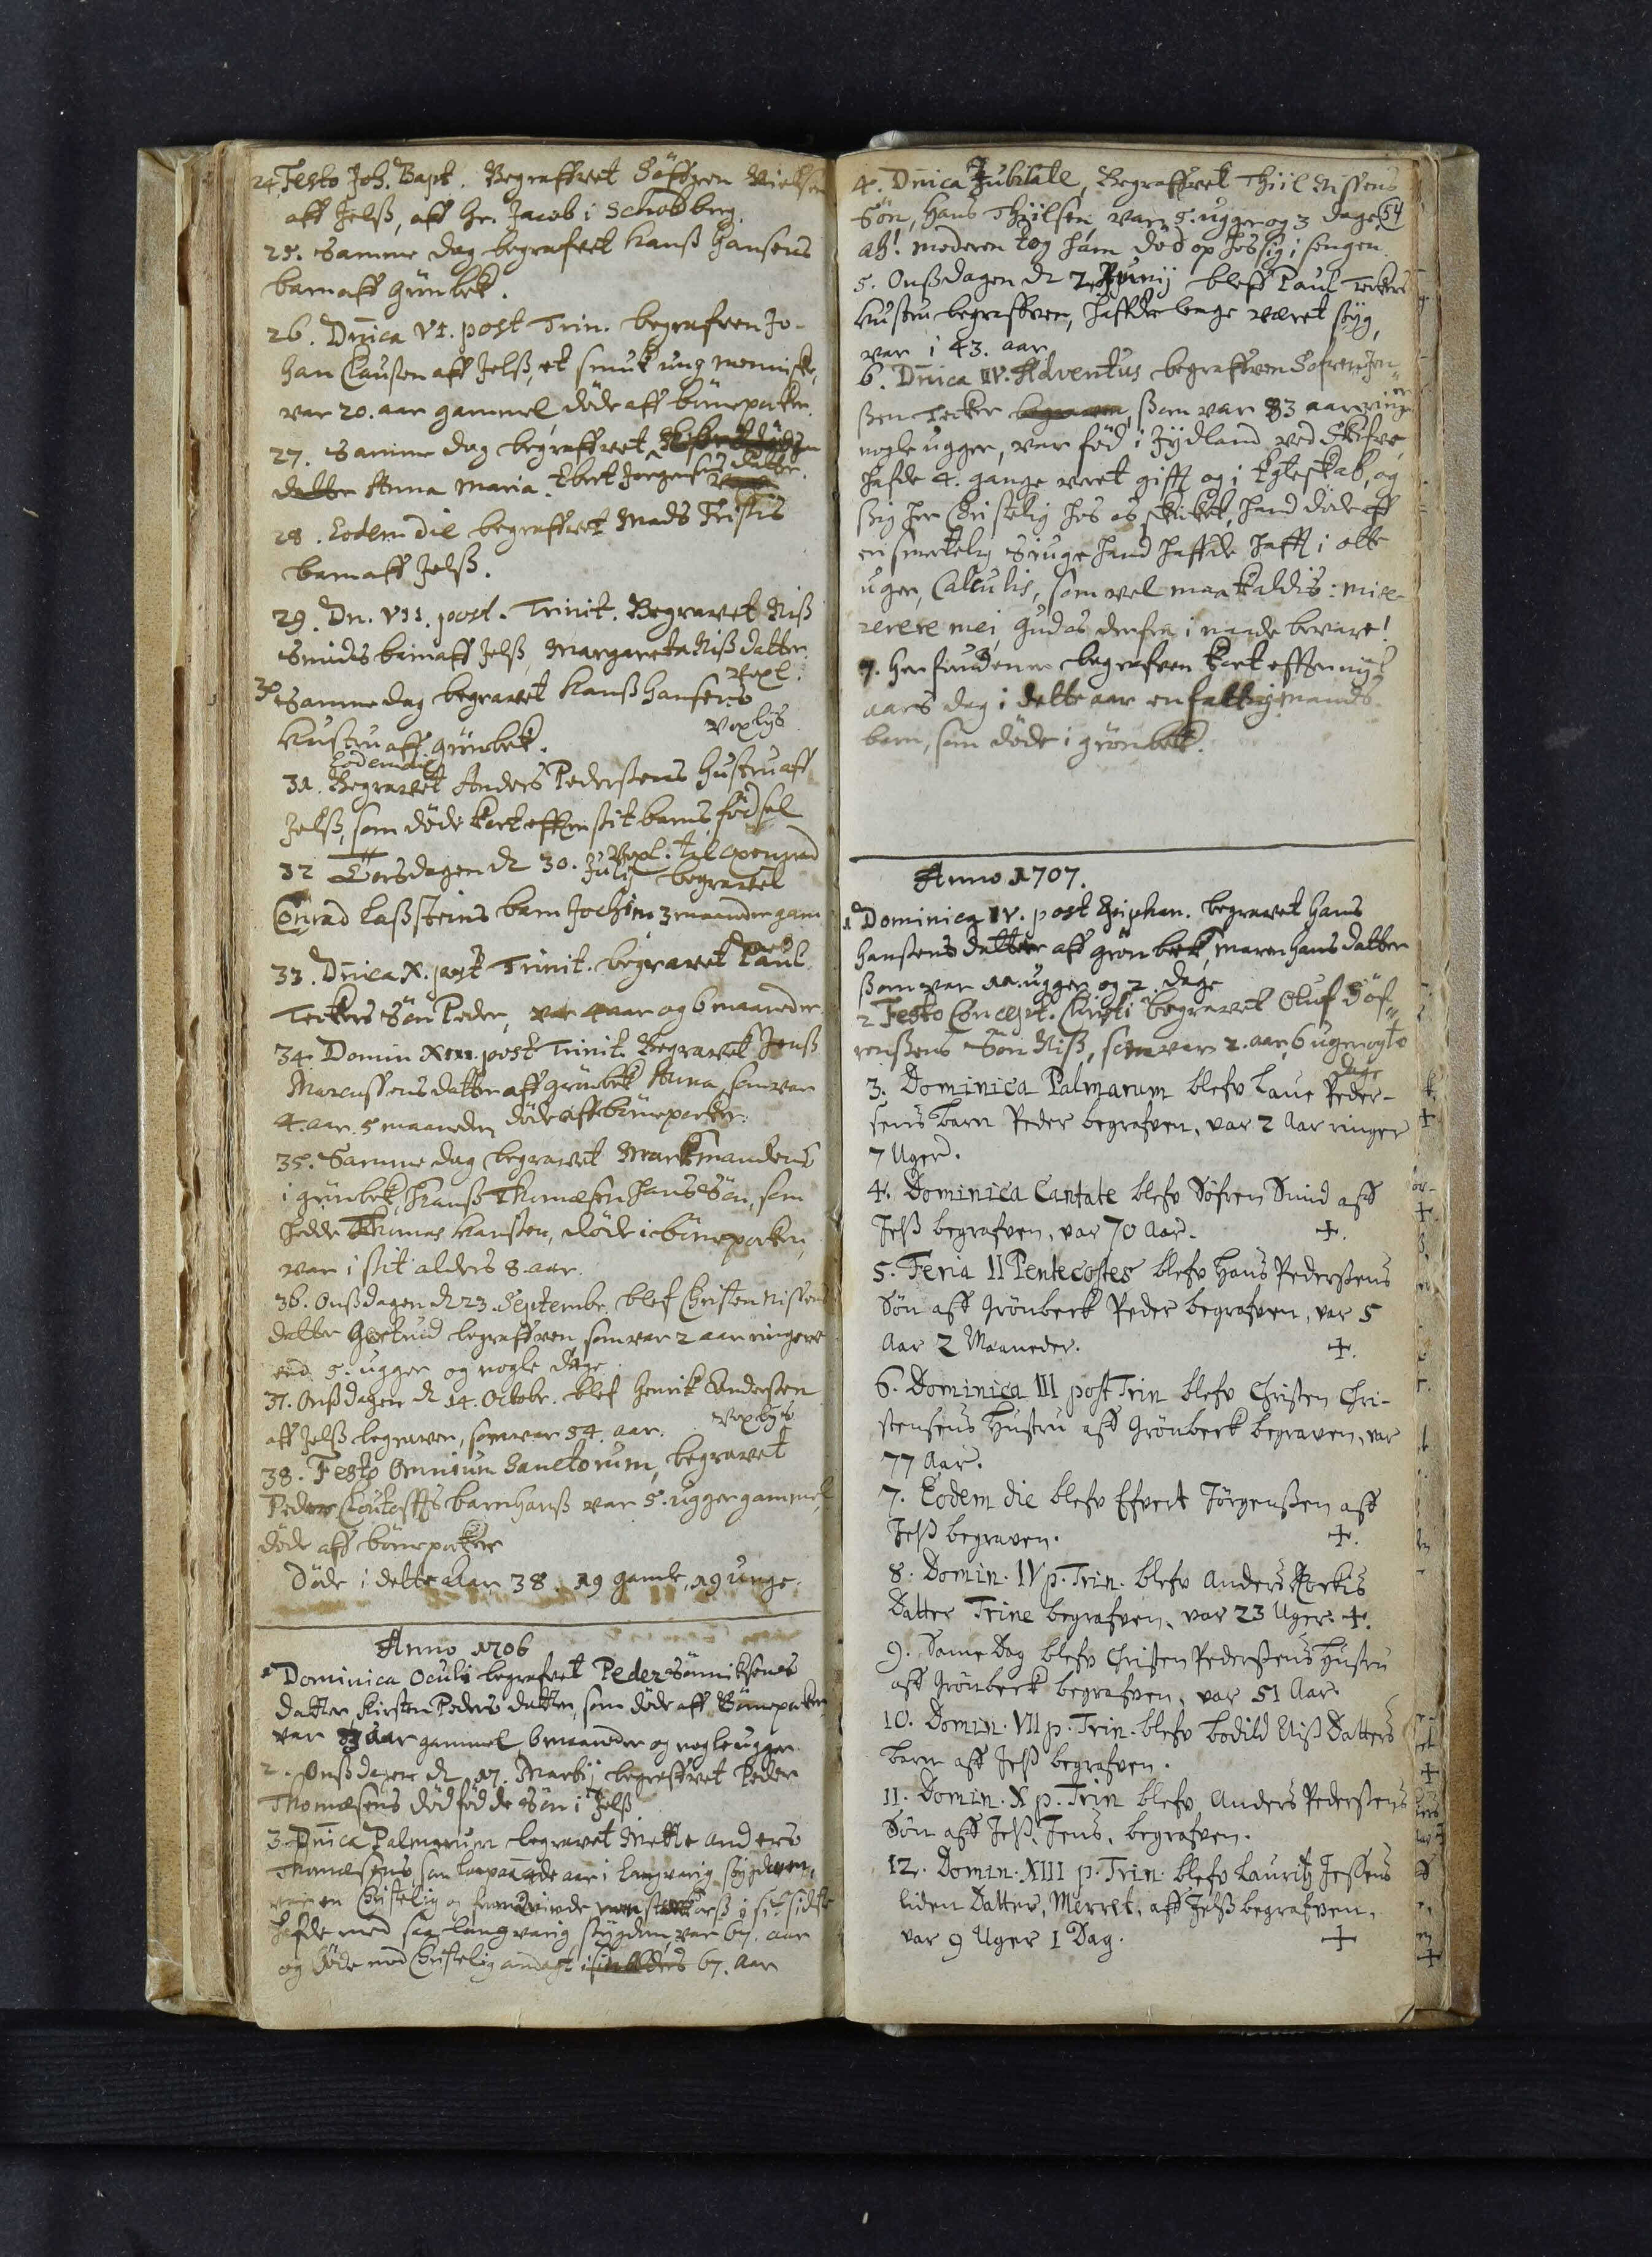24. Festo Joh. Bapt. Begraffvet Søffren Nielsen
aff Jels, aff Hr. Jacob i Schodborg
25. Samme Dag begrafvet Hanss Hansens
barn aff Grønbek.
26. Dnica VI. post Trin. begrafven Jo¬
han Claussen aff Jelss, et smuk ung menniske,
var 20. aar gammel, døde aff børnepocker.
27. Samme dag begraffvet Ebert Jørgensens
datter Anna Maria. 
Ebert Jørgensens datter
28. Eodem die begraffvet Mads Frisis
barn aff Jelss.
29. Dn. VII. post Trinit. Begravet Niss
Smids barn aff Jelss, Margaretha Niss Datter.
Woxl.
30. Samme dag begravet Hanss Hansens
Hustru aff Grønbek.
Woxlys
Eodem die
31. begravet Anders Pederssens Hustru aff
Jelss, som døde kort effter sit barns fødsel
Woxl. Til Oxenvad
32. Torsdagen d 30. Julij begravet
Conrad Lassteins barn Jochim 3 maaneder gam
33. Dnica X. post Trinit. begravet Paul
Teckers søn Peder, var 4 aar og 6 maaneder
34. Domin XIII. post Trinit. begravet Jenss
Marcussens datter aff Grønbek Anna, som var
4. aar. 5 maaneder døde aff børnepocker
35. Samme dag begravet Markmandens 
i Grønbek, Hans Thomæsen hans Søn, som 
hedde Thomas Hansen, døde aff børnepocker,
var i ssit alders 8. aar
36. Onssdagen d 23. Septembr. blef Christen Nissens
datter Gertrud begraffven som var 2 aar ringere
end 5. ugger og nogle dage
37. Onssdagen d 14. Octobr. blef Henrik Anderssen
aff Jelss Begraven, som var 34. aar. Woxlys
38. Festo Omnium Sanctorum , begravet
Peder Cloutoffts barn Hanss var 5. ugger gammel,
døde aff børnepocker
Døde i dette Aar 38. 19 gamle, 19 unge
Anno 1706
1. Dominica Oculi begrafvet Peder Sønnicksens
datter, Kirsten Peders Datter, som døde aff Børnepocker,
var 3 Aar gammel 6 maaneder og nogle ugger
2. Onssdagen d 17. Martij begraffvet Peder
Thomæsens dødfødde søn i Jelss
3. Dnica Palmarum begravet Mette Anders
Thomæsens, som laa paa 4de aar i langvarig sygdom,
var en Christelig og from Qvinde men st##ess i sit sidste
hafde med saa langvarig sygdom, var 67. aar
og døde med Christelig andakt i sin alders 67. Aar.
54
4. Dnica Jubilate , Begraffvet Thiil Nissens
søn, Hans Thiilsen, var 5. ugger og 3 dage,
ah! Moderen tog ham død op hos sig i sengen.
5. Onssdagen d 2. Junij bleff Paul Teckers
Hustru begraffven, hafde lenge været syg,
var i 43. aar
6. Dnica IV. Adventus begraffven Søfren Jen¬
ssen Tecker ##, som var 83 aar ringer
nogle ugger, var fød i Jydland ved Skifve,
hafde 4. gange veret gifft og i Egteskab, og
ssig her Christelig hos os skicket, hand døde aff
en smertelig Siuge hand haffde haft i otte
uger, Caleulis, som vel maa kaldis: mise
rerere mei, Gud os derfra i naade bevare
7. Her funden## begrafven kort effter nyt
aars dag i dette aar en fattig mands
barn, som døde i Grønbek.
Anno 1707.
1. Dominica IV. post Epiphan. begravet Hans
Hanssens datter aff Grønbeck, Maren Hans datter
ssom var 11. ugger og 2. dage
2. Festo Concept. Christi begravet Oluf Søf¬
renssens Søn Niss, som var 2. aar, 6 uger og to
Dage
3. Dominica Palmarum blefv Laue Peder¬
sens barn Peder begrafven, var 2 Aar ringer
7 Uger.
4. Dominica Cantate blefv Søfren Smid aff
Jelss begrafven, var 70 Aar.
5. Feria II Pentecostes blefv Hans Pederssens
Søn aff Grønbeck Peder begrafven, var 5
Aar 2 Maaneder.
6. Dominica III post Trin blefv Christen Chri¬
stensens Hustru aff Grønbeck begraven, var
77 Aar.
7. Eodem die blefv Efvert Jørgenssen aff
Jels begraven.
8. Domin. IV p. Trin. blefv Anders Kockis
Datter Trine begrafven, var 23 Uger.
9. Same Dag blefv Christen Pederssens Hustru
aff Grønbeck begrafven, var 51 Aar.
10. Domin. VII p. Trin. blefv Bodild Niss Datters
barn aff Jels begrafven
11. Domin. X p. Trin. blefv Anders Pedersens
Søn aff Jels, Jens, begrafen.
12. Domin. XIII p. Trin. blefv Lauritz Jessens
liden Datter, Merret, aff Jelss begrafven,
var 9 Uger 1 Dag.
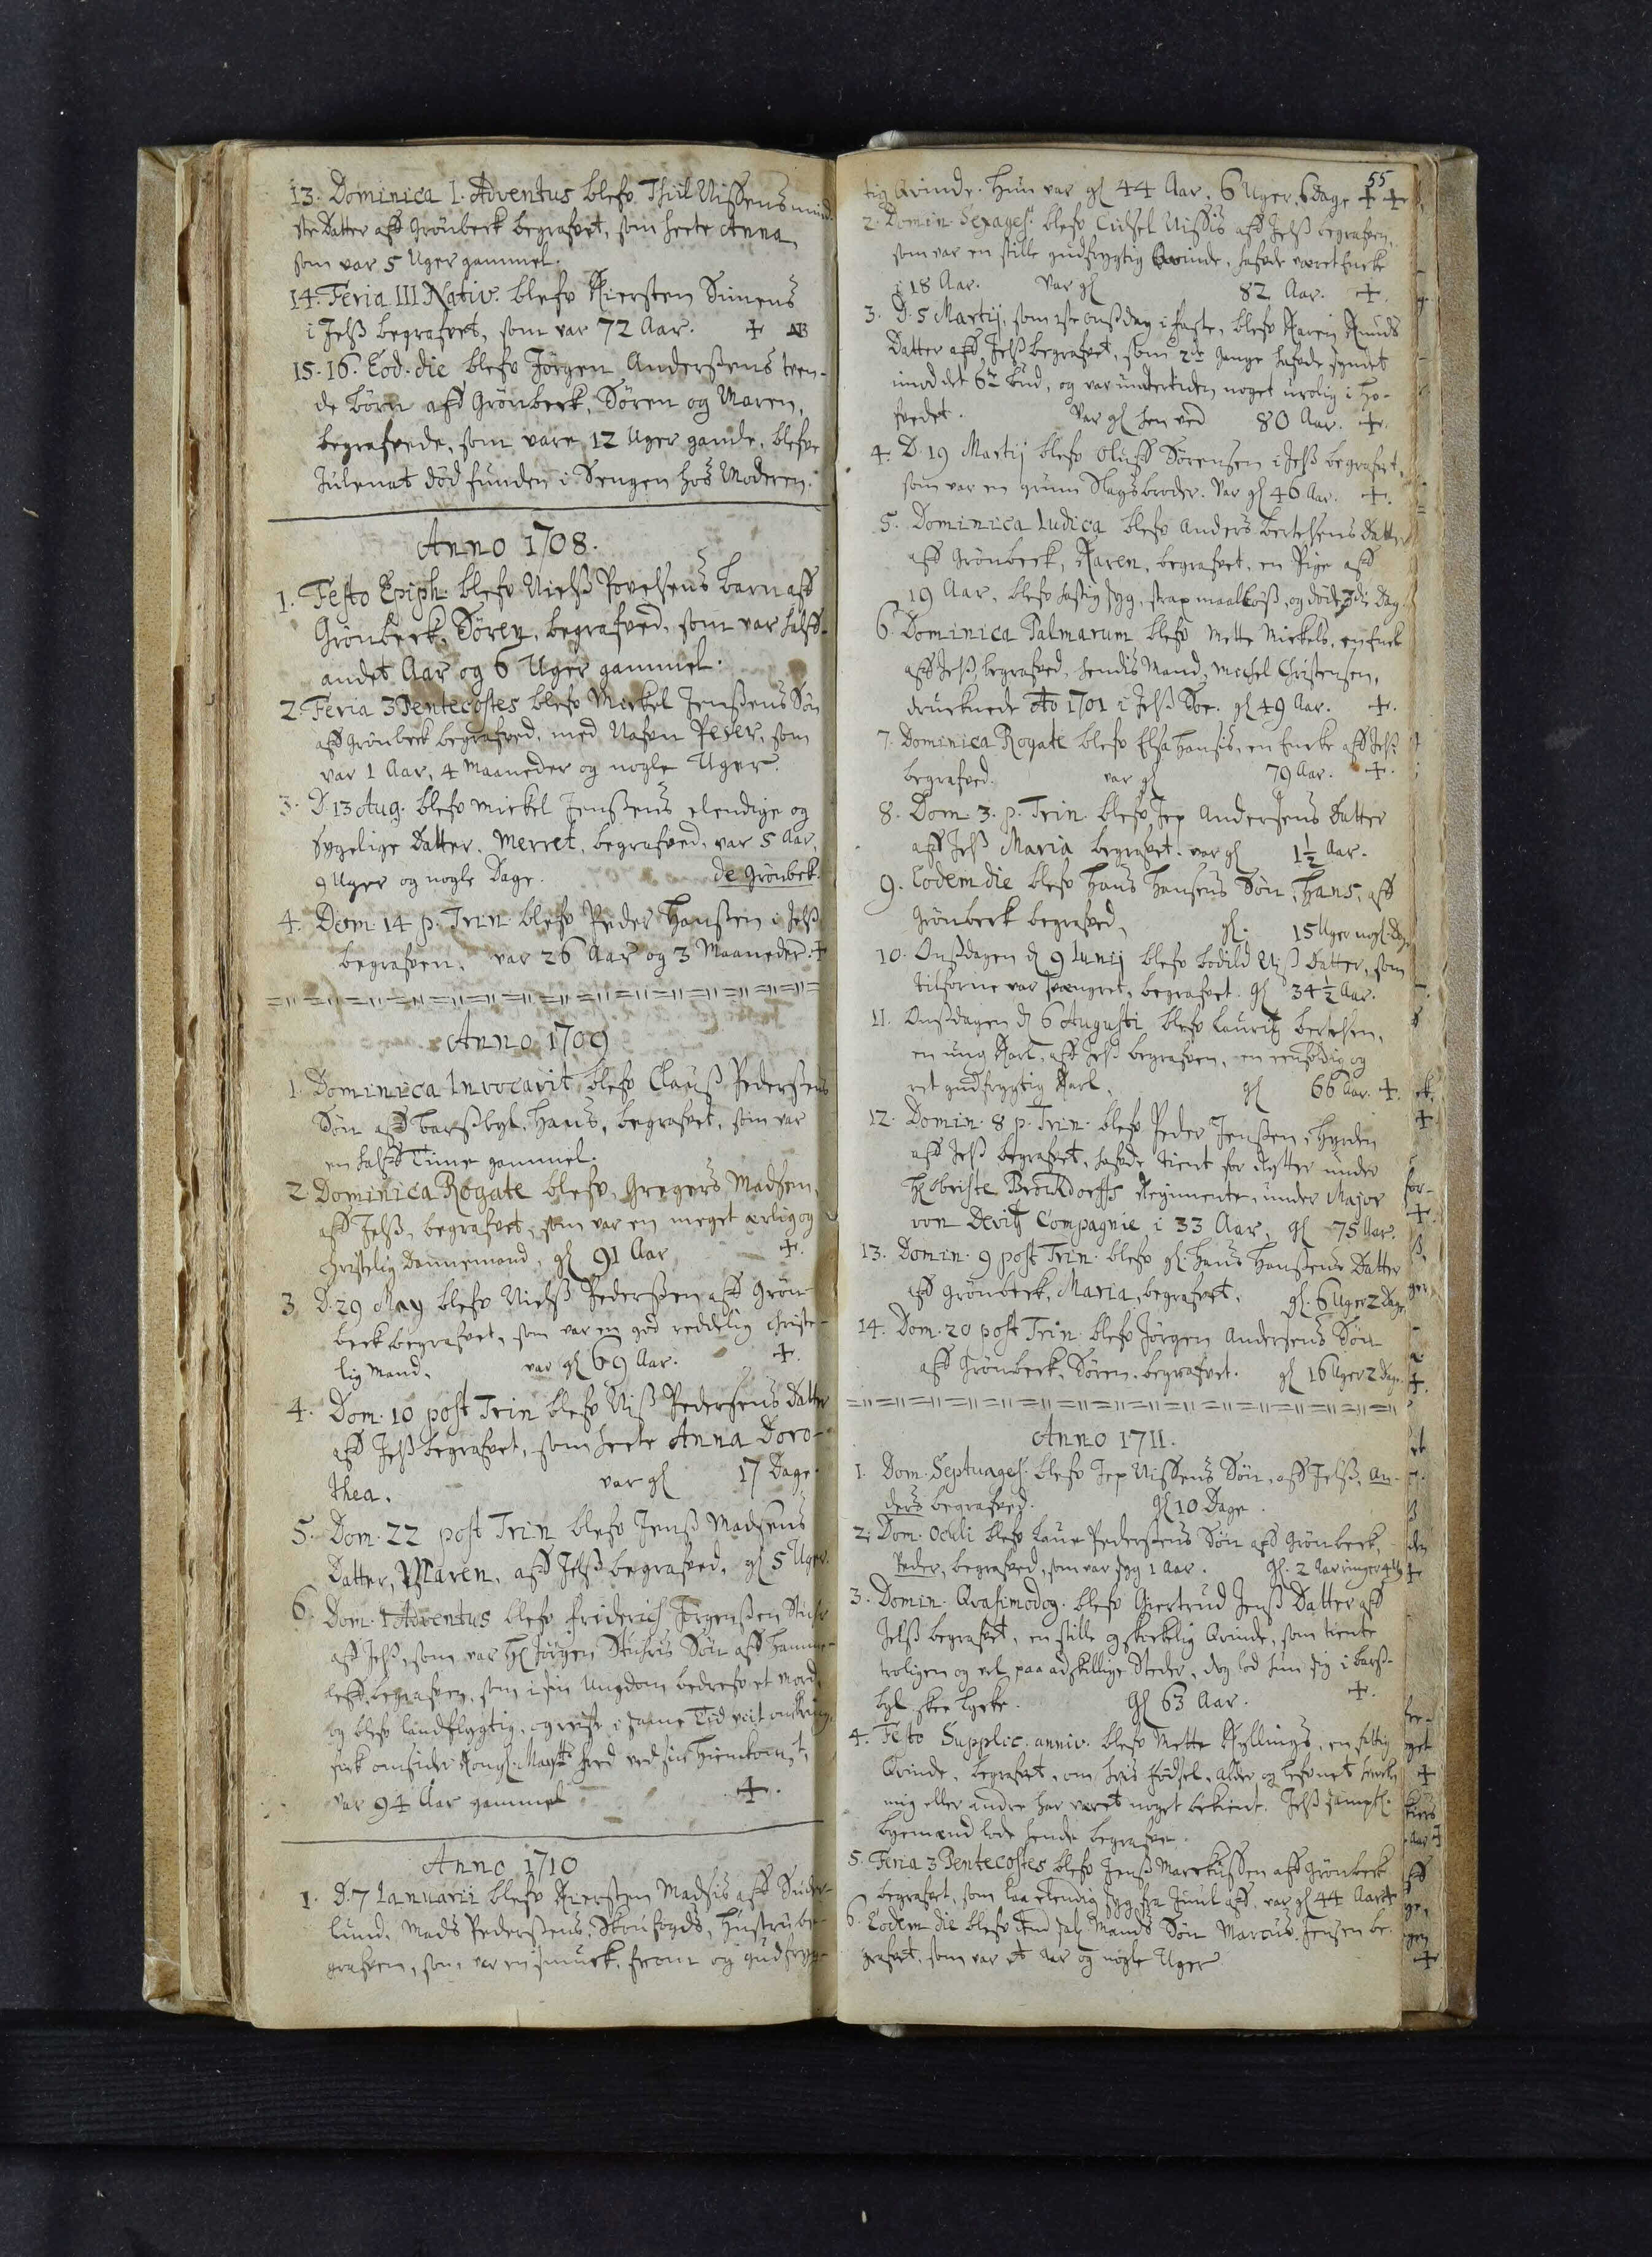13. Dominica 1. Adventus blefv Thiil Nissens mind¬
ste Datter aff Grønbeck begrafvet, som heete Anna,
som var 5 Uger gammel
14. Feria III Nativ. blef Kiersten Simens
i Jelss begrafvet, som var 72 Aar.
15. 16. Eod. die blefv Jørgen Anderssens tven
de børn aff Grønbeck, Søren og Maren, 
begrafvede, som var 12 Uger gamle, blefve
Julenat død funden i Sengen hos Moderen.
Anno 1708
1. Festo Epiph. blefv Nielss Povelsens barn aff
Grønbeck, Søren, begrafved, som var halff
andet Aar og 6 Uger gammel.
2. Feria 3 Pentecostes blefv Mickel Jenssens Søn
aff Grønbeck begrafved, med Nafvn Peder, som
var 1 Aar, 4 Maaneder og nogle Uger
3. D 13 Aug. Blefv Mickel Jenssens elendige og
sygelige Datter, Merret, begrafved, var 5 Aar,
9 Uger og nogle Dage. de Grønbek.
4. Dom. 14 p. Trin. blefv Peder Hanssen i Jelss
begrafven, var 26 Aar og 3 Maaneder.
Anno 1709
1. Dominica Invocavit blefv Clauss Pederssens
Søn aff Barssbyl, Hans, begrafvet, som var
en halff Time gammel.
2. Dominica Rogate blefv Gregers Madsen
aff Jelss, begrafvet, som var en meget arlig og
christelig Dannemand. gl 91 Aar
3. D. 29 May blefv Nielss Pederssen aff Grøn¬
beck begrafvet, som var en god reddelig christe¬
lig Mand. var gl 69 Aar.
4. Dom. 10 post Trin blefv Niss Pedersens Datter
aff Jelss begrafvet, som heete Anna Doro¬
thea.
var gl. 17 Dage.
5. Dom. 22 post Trin blefv Jenss Madsens
Datter, Maren, aff Jelss begrafved. gl 5 Uger.
6. Dom. 1 Adventus blefv Friderich Jørgenssen Stuhr
aff Jelss, som var Hl Jørgen Stuhris Søn aff Hamme¬
leff, begrafven, som i sin Ungdom bedrefv et mord,
og blefv landflygtig, og reiste i hans Tid veit omkring
fick omsider Kongl. Maystts fred ved sin Hiemkomst,
var 94 Aar gammel
Anno 1710
1. D. 7 Ianuarii blefv Kiersten Madsis aff Suder¬
lund, Mads Pederssens, Skoufogds, Hustru be¬
grafven, som var en smuck, from og gudfryg¬
55
tig Qvinde. Hun var gl 44 Aar, 6 Uger, 6 dage
2. Domin. Sexages. blefv Cidsel Nissis aff Jelss begrafven,
som var en stille gudfrygtig Qvinde, hafvde været Encke
i 18 Aar. Var gl
82 Aar.
3. D. 5 Martij, som ##e onssdag i Faste, blefv Karin Knuds
Datter aff Jelss begrafvet, som 2de gange hafvde syndet
imod det 6te bud, og var undertiden noget urolig i Ho¬
fvedet.
var gl hen ved 80 Aar.
4. D 19 Martij blefv Oluff Sørensen i Jelss begrafvet,
som var en grum Slagsbroder. Var gl 46 Aar.
5. Dominica Iudica blefv Anders Bertelsens Datter
aff Grønbeck, Karen, begrafvet, en Pige aff
19 Aar. blef hastig syg, strax maalløss, og døde 3die Dag.
6. Dominica Palmarum blefv Mette Mickels, en Encke
aff Jelss begrafved, hendis Mand, Mickel Christensen,
druknede Ao 1701 i Jelss Søe. gl 49 Aar.
7. Dominica Rogate blefv Elsa Hansis, en Encke aff Jelss
begrafved.
var gl
79 Aar.
8. Dom. 3. p. Trin blefv Jep Andersens Datter
aff Jelss Maria begrafvet. var gl 1½ Aar.
9. Eodem die blefv Hans Hansens Søn, Hans, aff
Grønbeck begrafved,
gl. 15 Uger nogl Dage
10. Onssdagen d 9 Iunij blefv Bodild Niss Datter, som
tilforne var svangret, begrafet. gl 34½ Aar.
11. Onssdagen d 6 Augusti blefv Lauritz Bertelsen,
en ung Karl aff Jelss begrafven, en eenfoldig og
ret gudfrygtig Karl
Gl. 66 Aar.
12. Domin. 8 p. Trin. blefv Peder Jenssen , Hyrden
aff Jelss begrafvet, hafvde tient for Rytter under
Hl Obriste Brockdorffs Regimente under Major
von Devitz Compagnie i 33 Aar, gl 75 Aar.
13. Domin. 9 post Trin blefv gl. Hans Hanssens Datter
aff Grønbeck, Maria, begrafvet. gl 6 Uger 2 Dage
14. Dom. 20 post Trin blefv Jørgen Andersens Søn
aff Grønbeck, Søren, begrafvet. gl 16 Uger 2 Dage
Anno 1711.
1. Dom. Septuages. Blef Jep Nissens Søn aff Jelss, An¬
ders begrafved.
gl 10 Dage
2. Dom. Oculi blef Laue Pederssens Søn aff Grønbeck,
Peder, begrafved, som var syg 1 Aar. gl. 2 Aar ringer 4 Ug
3. Domin. Qvasimodog. blefv Giertrud Jenss Datter aff
Jelss begrafvet, en stille og skickelig Qvinde, som tiente
troligen og vel paa adskillige Steder, dog lod hun sig i Barss
byl skee Lycke.
gl 63 Aar.
4. Festo Supplic. Anniv. blefv Mette Kyllings, en fattig
Qvinde, begrafet, om hvis fødsel, alder og lefnet hverken
mig eller andre har været noget bekiende. Jelss samptl.
byemænd lod hende begrafve
5. Feria 3 Pentecostes blefv Jenss Marckussen aff Grønbeck
begrafvet, som laa elendig syg fra Juul aff, var gl 44 Aar
6. Eodem die blefv dend sal. Mands Søn Marcus Jessen be¬
grafvet, som var et Aar og nogle Uger